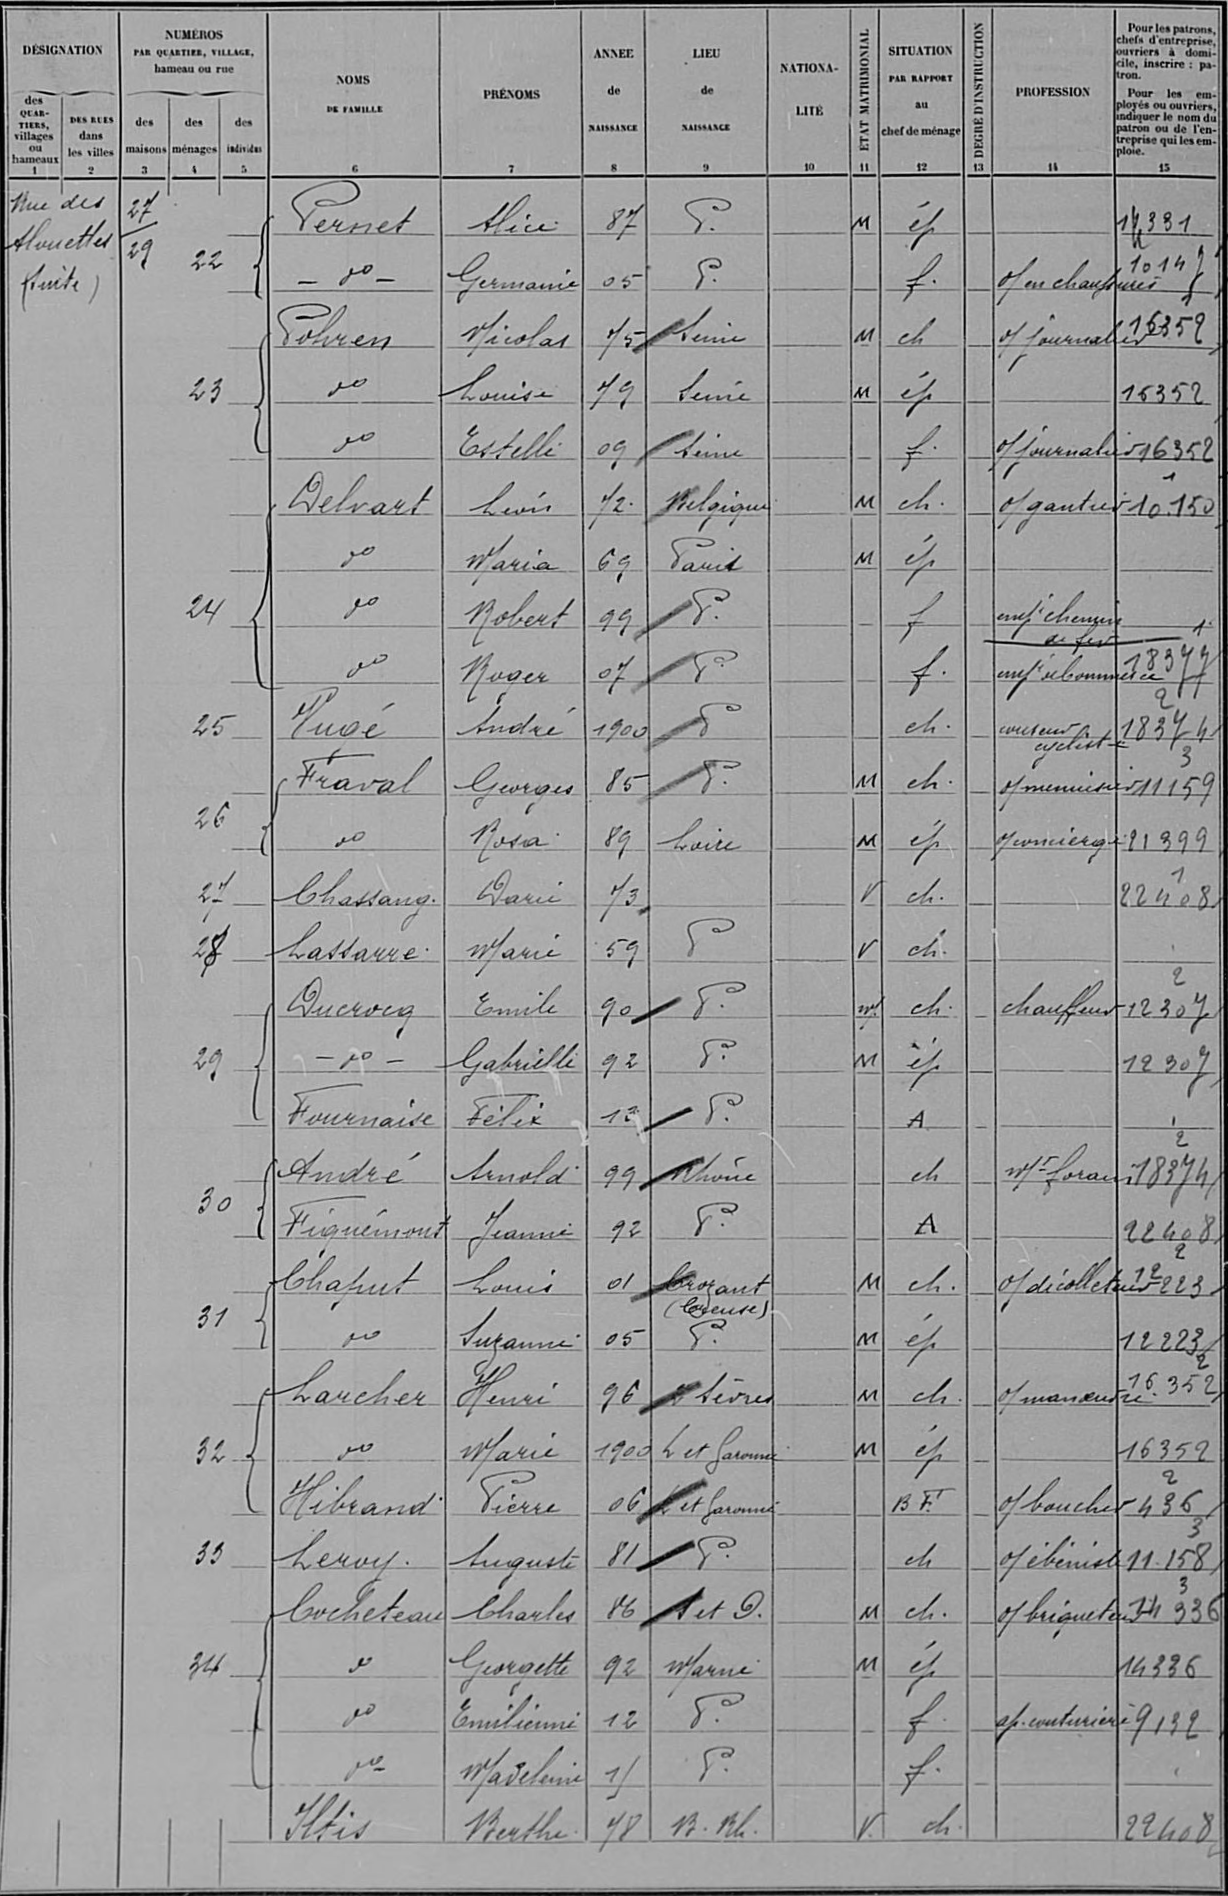Peusset/Alice/87/P /¤/M/ép/¤/¤/14331
d°/Germanie/05/P /¤/¤/f /¤/o en chaussures/10149
Pohren/Nicolas/75/Seine/¤/M/ch/¤/o journalier/16352
d°/Louise/79/Seine/¤/M/ép/¤/¤/16352
d°/Estelle/09/Seine/¤/¤/f /¤/o journalier/16352
Delvaut/Louis/72/Belgique/¤/M/ch /¤/o gantier/10150
d°/Maria/69/Paris/¤/M/ép/¤/¤/¤
d°/Robert/99/P /¤/¤/f/¤/empl!chemin de fer/1
d°/Roger/07/P/¤/¤/f /¤/empl bonnetier/18377
Dugé/André/1900/P/¤/¤/ch /¤/coureur!cycliste/18374
Fraval/Georges/85/P/¤/M/ch /¤/o menuisier/11159
d°/Rosa/89/Loire/¤/M/ép/¤/o concierge/21399
Chassang/Dami/73/¤/¤/V/ch /¤/¤/22408
Lassanne/Marie/59/P/¤/V/ch/¤/¤/2
Ducrocq/Emile/90/P/¤/m/ch/¤/chauffeur/12307
d°/Gabrielle/92/P/¤/M/ep/¤/¤/12307
Fournaise/Félix/13/P /¤/¤/A/¤/¤/¤
André/Arnold/99/Rhone/¤/¤/ch/¤/m forain/18374
Friquémont/Jeanne/92/P /¤/¤/A/¤/¤/22408
Chafrut/Louis/01/Crozant!(Creuse)/¤/M/ch /¤/o decolleteur/12223
d°/Suzanne/05/P/¤/M/ép/¤/¤/12223
Laucher/Henri/96/2 Sèvres/¤/M/ch /¤/o manoeuvre/16352
d°/Marie/1900/L et Garonne/¤/M/ép/¤/¤/16352
Hibrand/Pierre/06/L et Garonne/¤/¤/BF/¤/o boucher/436
Leuvy/Auguste/81/P/¤/¤/ch/¤/o ébéniste/11158
Cocheteau/Charles/86/s et L/¤/M/ch /¤/o briqueteur/24936
d°/Georgette/92/Marne/¤/M/ép/¤/¤/14336
d°/Emilienne/12/P/¤/¤/f /¤/o couturière/9132
d°/Madeleine/15/P/¤/¤/f /¤/¤/¤
Iltis/Marthe/78/B Rh/¤/V/ch /¤/¤/22408
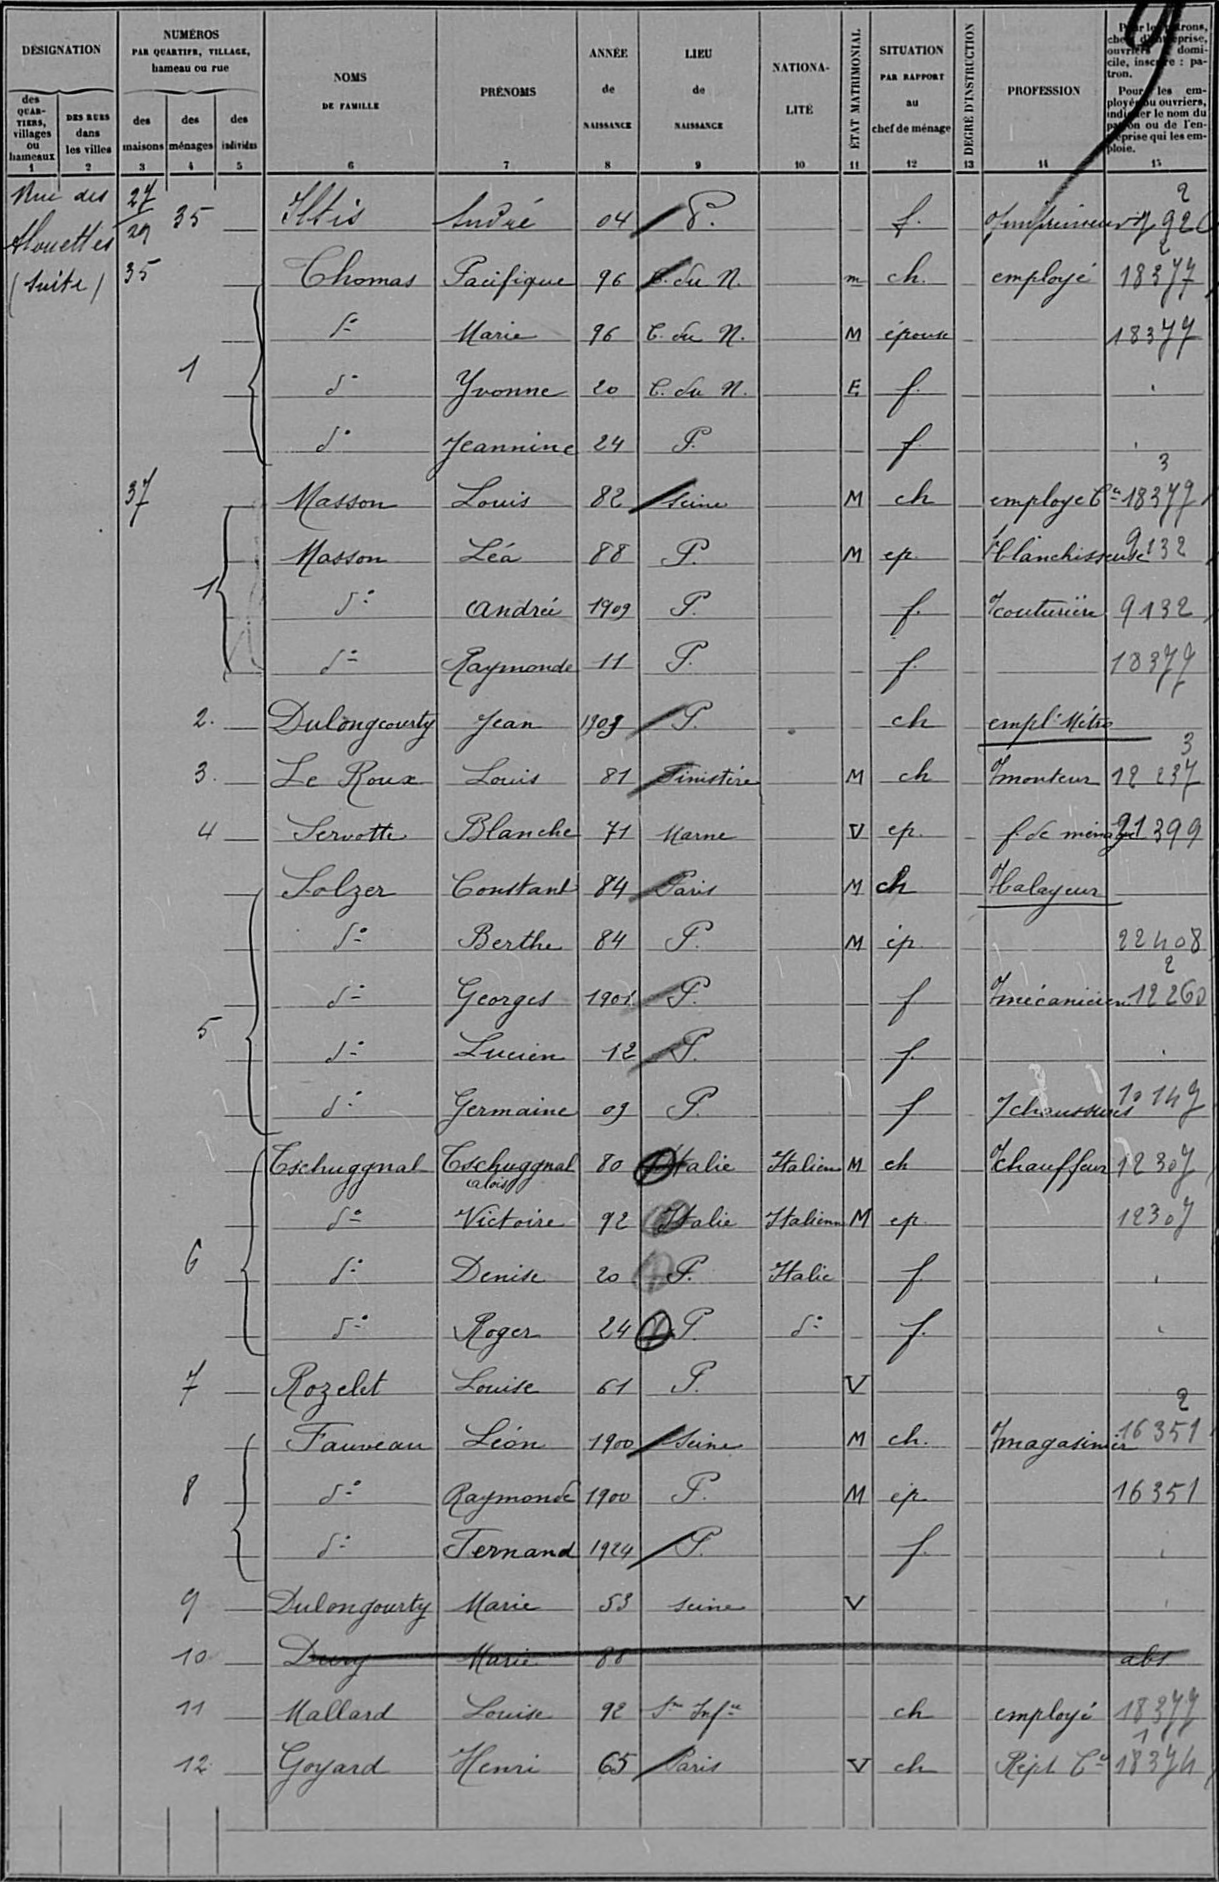Iltis/André/04/P /¤/¤/f /¤/o imprimeur/7920
Thomas/Pacifique/96/C du N /¤/m/ch /¤/employé/18377
d°/Marie/96/C du N /¤/M/épouse/¤/¤/18377
d°/Yvonne/20/C du N /¤/E/f /¤/¤/¤
d°/Jeannine/24/P /¤/¤/f/¤/¤/3
Masson/Louis/82/seine/¤/M/ch/¤/employé C/18377
Masson/Léa/88/P/¤/M/ep /¤/blanchisseuse/9132
d°/andrée/1909/P /¤/¤/f /¤/o couturière/9132
d°/Raymonde/11/P/¤/¤/f/¤/¤/18377
Dulongcourty/Jean/1903/P/¤/¤/ch/¤/empl Métro/¤
Le Roux/Louis/81/Finistère/¤/M/ch/¤/o monteur/12237
Servotte/Blanche/71/Marne/¤/V/ep/¤/f de ménage/21399
Solzer/Constant/84/Paris/¤/M/ch/¤/balayeur/¤
d°/Berthe/84/P/¤/M/ep/¤/¤/22408
d°/Georges/1901/P/¤/¤/f/¤/o mécanicien/12260
d°/Lucien/12/P/¤/¤/f/¤/¤/¤
d°/Germaine/09/P/¤/¤/f/¤/o chaussures/10147
Tschuggnal/Tschuggnal!alois/80/Italie/Italien/M/ch/¤/chauffeur/12307
d°/Victoire/92/Italie/Italienne/M/ep/¤/¤/12307
d°/Denise/20/P /Italie/¤/f/¤/¤/¤
d°/Roger/24/P /d°/¤/f/¤/¤/¤
Rozelet/Louise/61/P /¤/V/¤/¤/¤/2
Fauveau/Léon/1900/seine/¤/M/ch/¤/magasinier/16351
d°/Raymonde/1900/P/¤/M/ép/¤/¤/16351
d°/Fernand/1924/P/¤/¤/f/¤/¤/¤
Dulongourty/Marie/53/seine/¤/V/¤/¤/¤/¤
Ducry/Marie/88/¤/¤/¤/¤/¤/¤/abs
Mallard/Louise/92/S inf/¤/¤/ch/¤/employé/18377
Goyard/Henri/65/Paris/¤/V/ch/¤/Rept C/18374